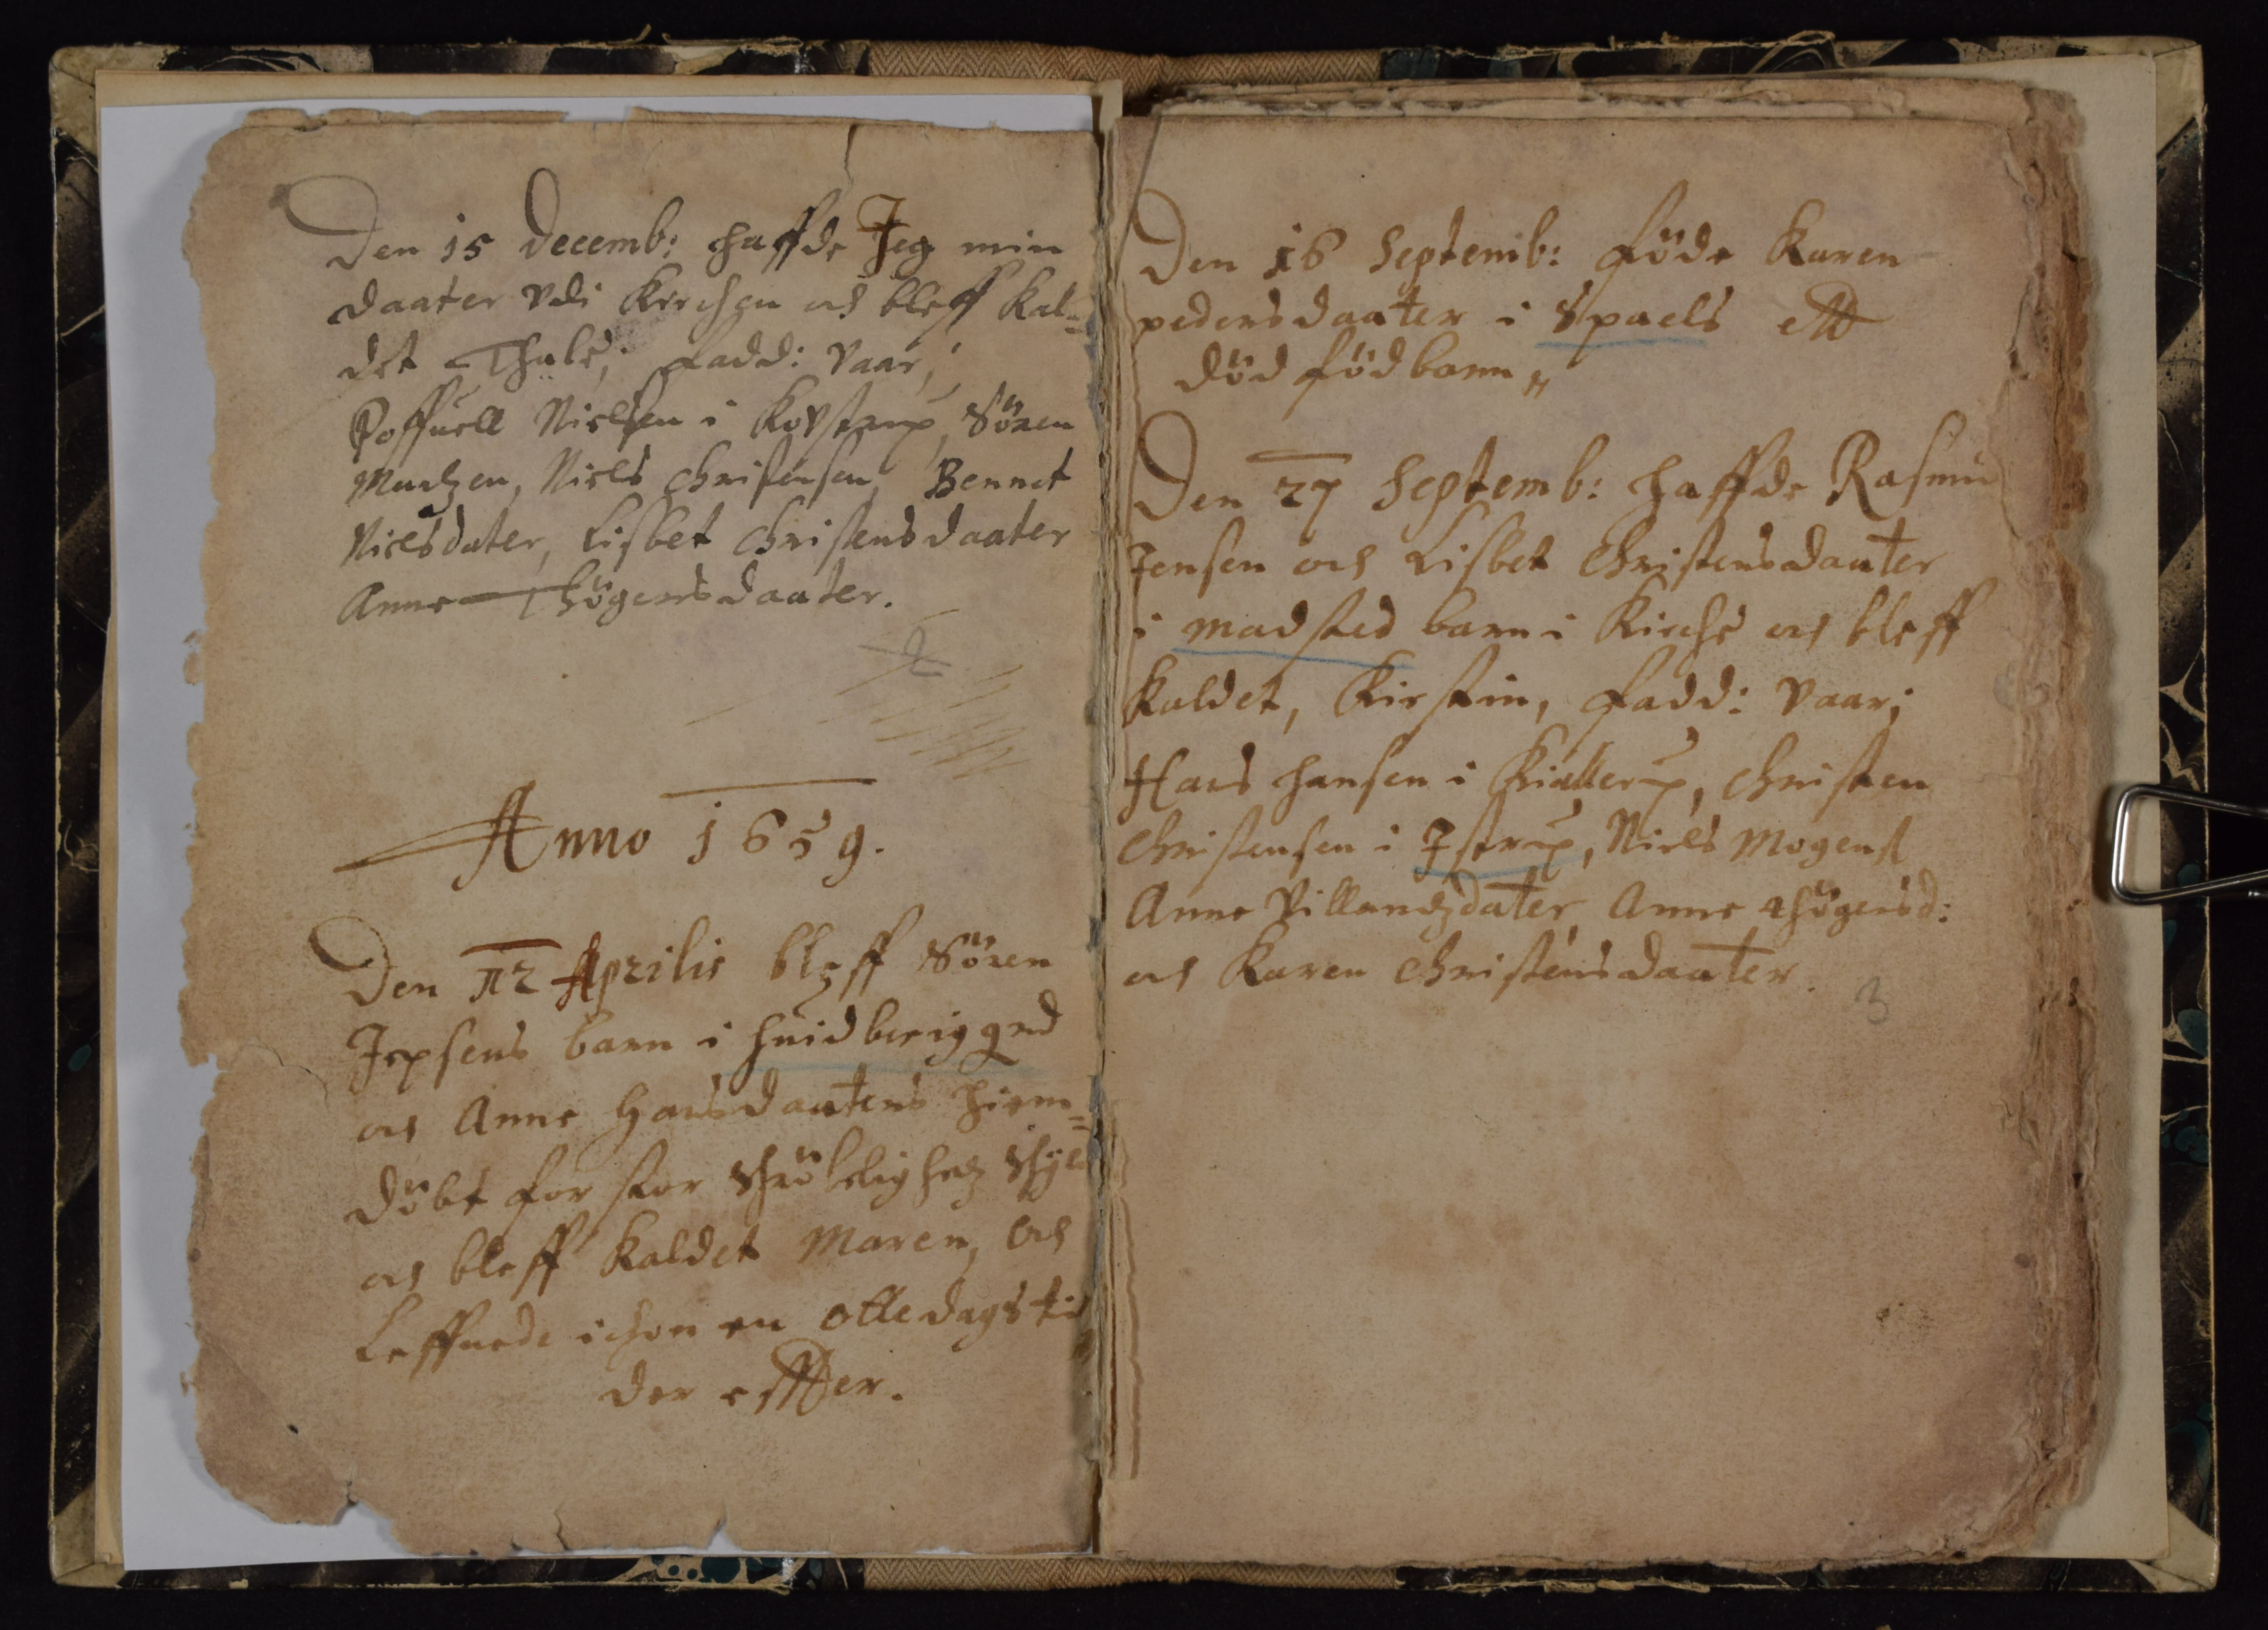Den 15 Decemb: haffde Jeg min
daater vdi Kirchen ud bleff kal¬
det Thale Fadd: vaar
Poffuell Nielsen i Kovstrup Sønen
Madzen, Niels Christensen, Bennet
Nielsdater, Lisbet Christensdaater
Anne Høgers daater
Anno 1659.
Den 12 Aprilis bleff Søren
Jepsens barn i Hvidberiggrd
och Anne Hansdauters Hiem¬
Døbt for stor schrøbelihhed schyld
ochs bleff kaldet Maren, och
leffuede ichon en otte dags tid
der effter.
Den 16 Septemb: føde Karen
Pedersdaater i Spaels ett
død fød barn
Den 27 Septemb: Haffde Rasmus
Jensen och Lisbet Christensdauter
i madsted barn Kirche och bleff
kaldet, Kirstin, Fadd: vaar;
Hans Hansen i Kiallerup, Christen
Christensen i Istrup, Niels Mogens
Anne Villandzdater Anne Thøgersd:
och Karen Christensdaater
3
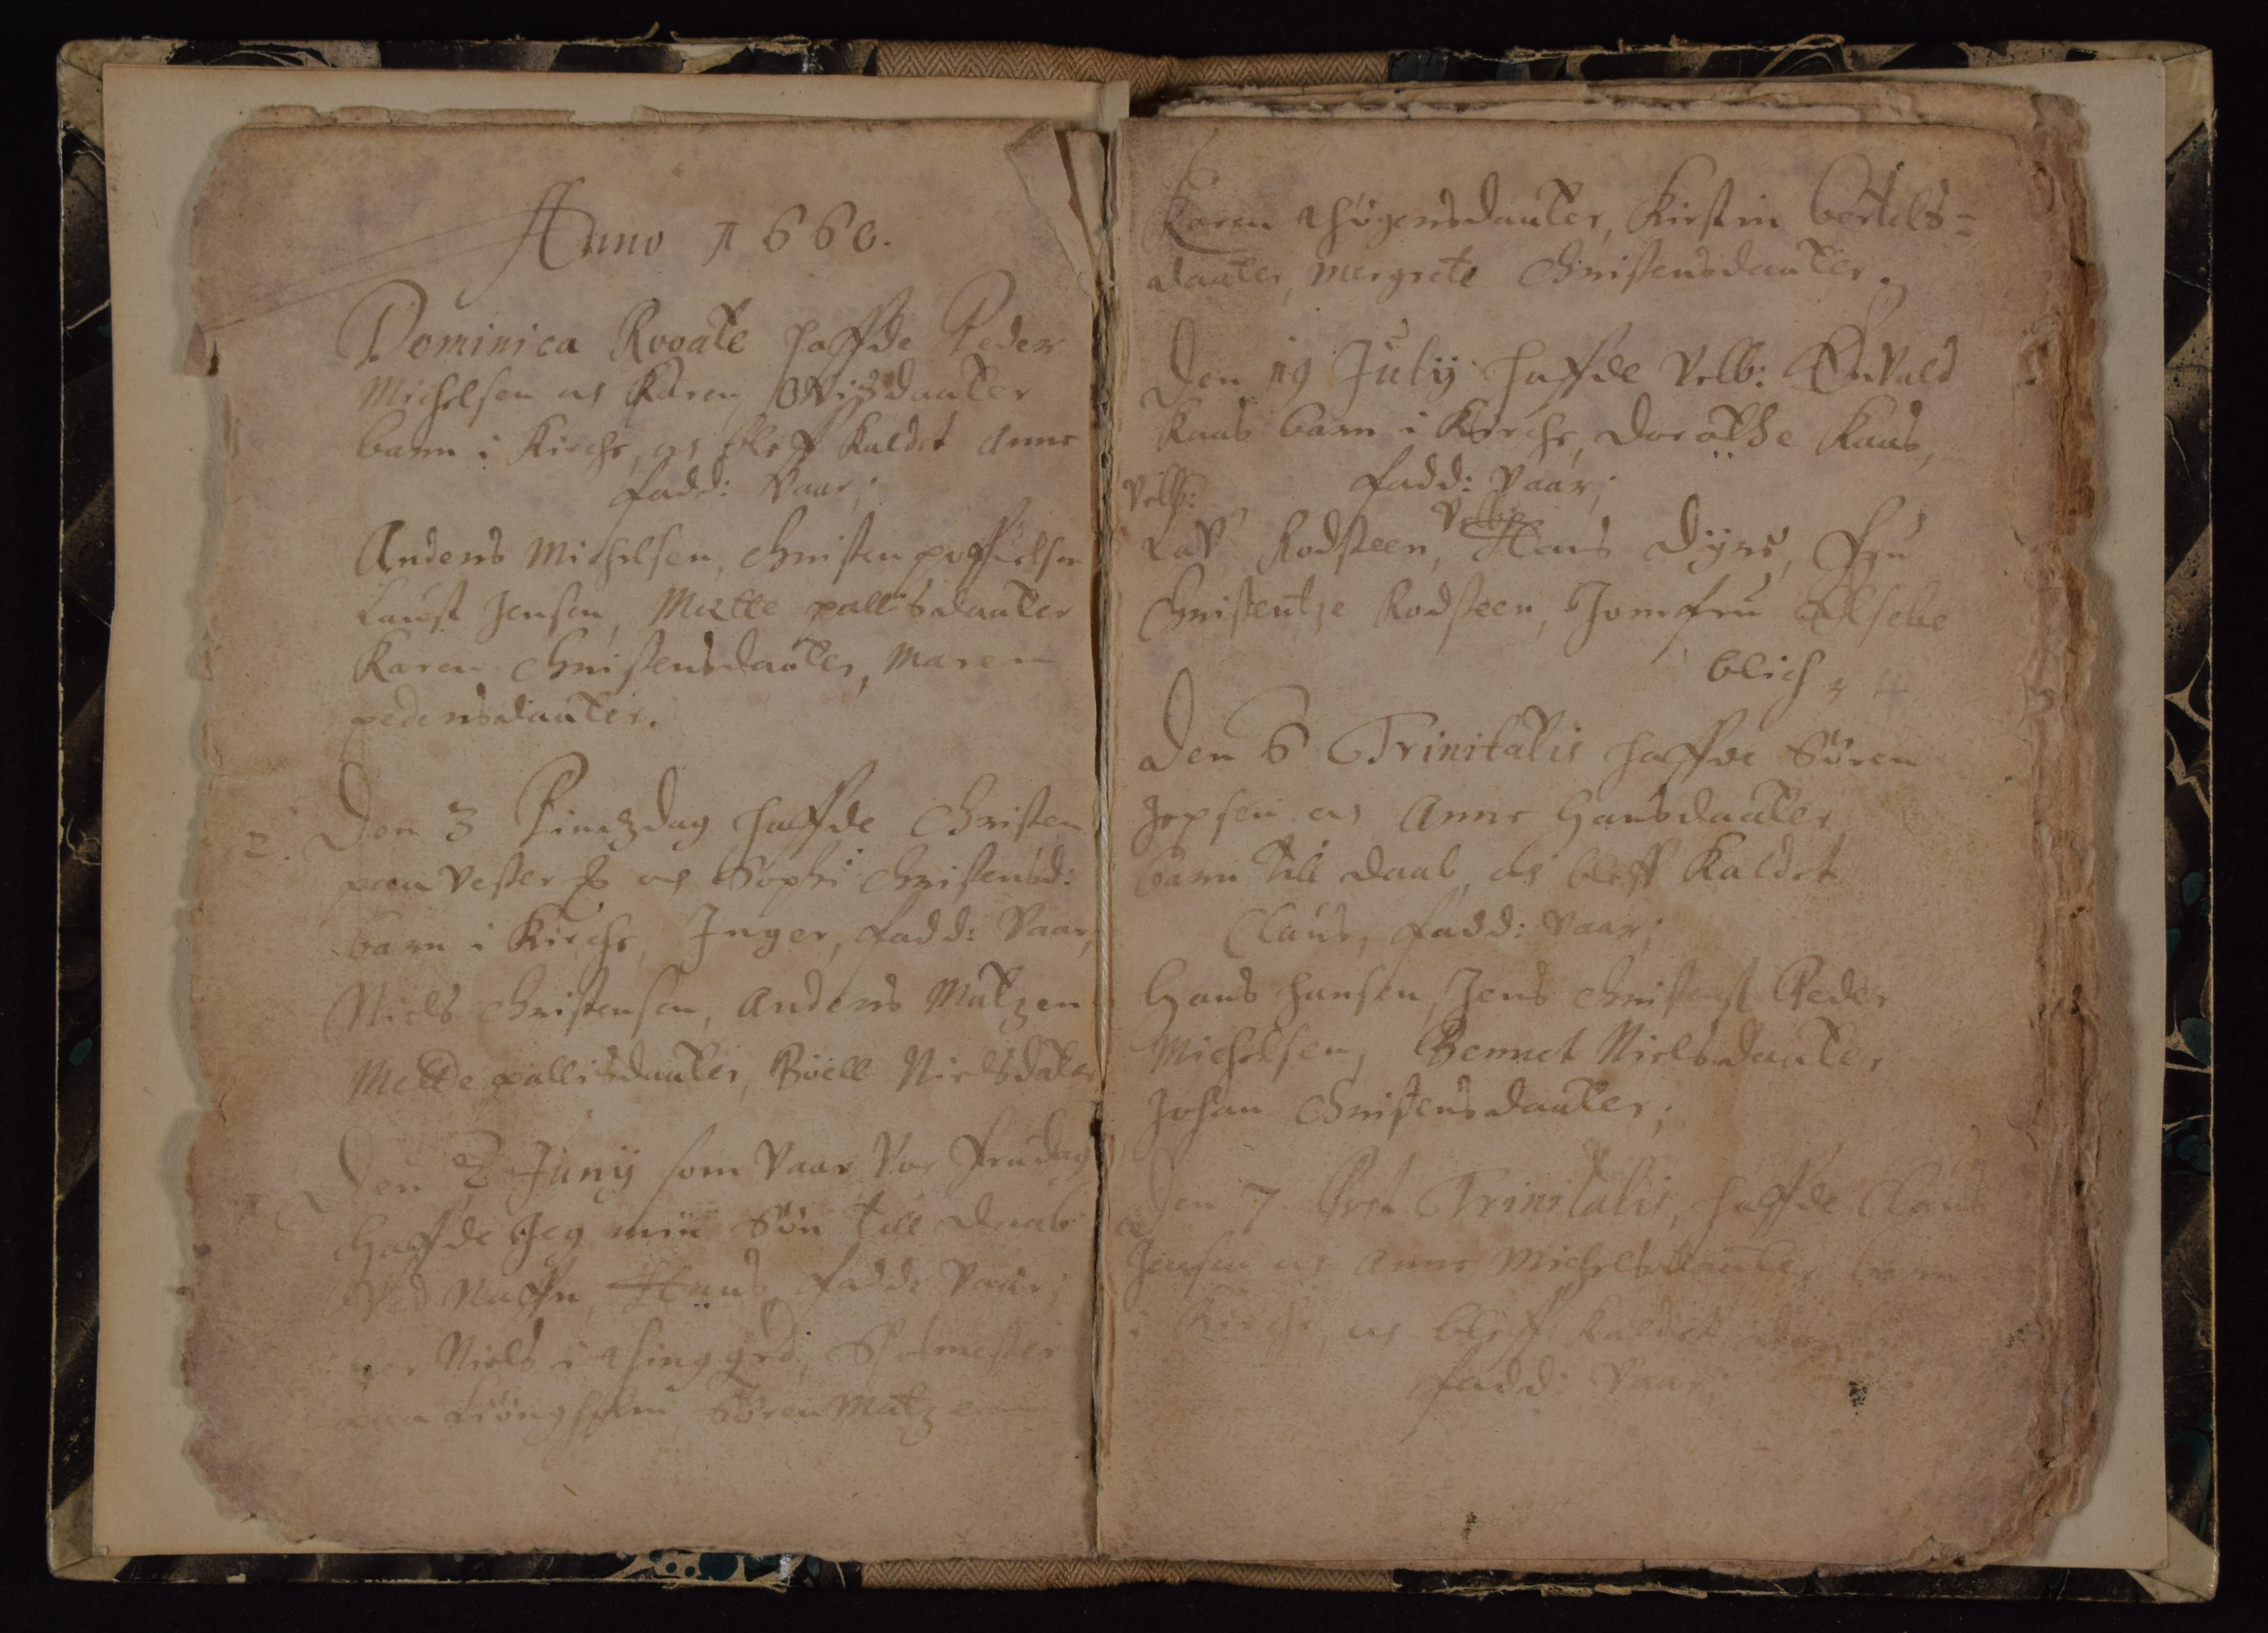Anno 1660.
Dominica Rogate haffde Peder
Michelsøn och Karen Ovisdaater
barn i Kirche, och bleff kaldet Anne
Fadd: vaar;
Anders Michelsen, Christen Poffuelsen
Laust Jensen Mætte pallisdaater
Karen Christensdaater Maren
Pedersdaater.
Dom 3 Pindtzdag haffde Christen
paa Vester E och Sophi Christensd:
barn i Kirche Inger, Fadd: vaar
Niels Christensen, Anders Matzen
Mette pallisdauter, Boell Nielsdatter
Den: 2 Junij som vaar vor Frudag
haffde Jeg ibin Søn till Daab
ved Naffn Hans Fadd: Vaar
## Niels i thing grd Skolmester
## Liøngholm, Søren Matzen
Karen thøgersdaater, Kirstin Bertels¬
dauter Margrete Christensdauter.
Den 19 Julij haffde Velb: Envold
Kaas barn i Kirche Dorothe Kaas
Fadd: vaar
Velb:
Velb:
Las Radsteen Hans Dyrs, Fru
Christentze Radsteen, Jomfru Elsebe
blich
Den 6. Trinitatis haffde Søren
Jepsøn och Anne Hansdaater
barn till daab och bleff kaldet
Claus; Fadd: vaar
Hans Hansøn, Jens Christens Peder
Michelsen, Bennet Nielsdaater
Johan Christens daatter
Dem 7 Post Trinitatis haffde Claus
Jensen och Anne Michelsdaater  barn
i Kirche, och bleff kaldet Jø##
Fadd: vaar;